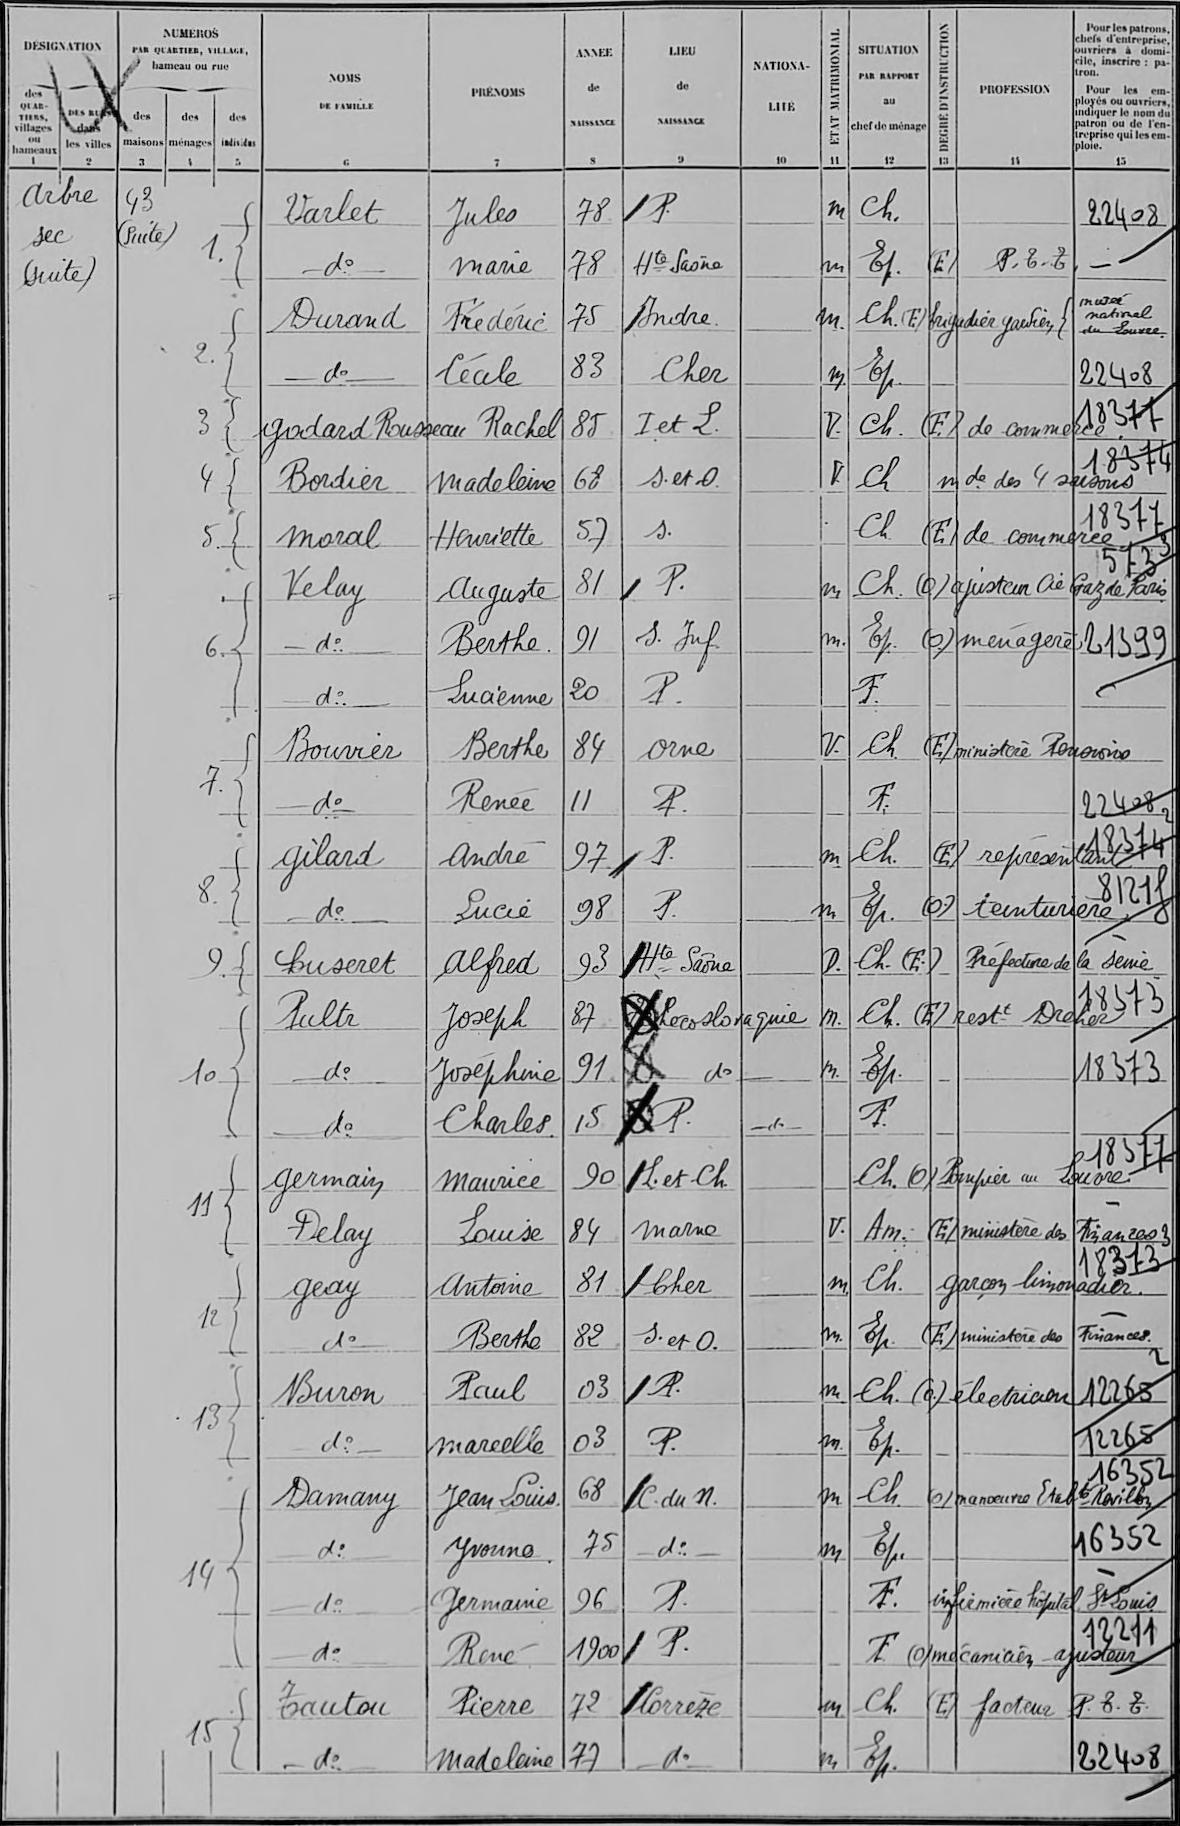Varlet/Jules/78/P/¤/M/Ch /¤/¤/22408
d°/marie/78/Hte Saône/¤/m/Ep/¤/(E) P T T /¤
Durand/Frédéric/75/Indre/¤/M/Ch /¤/(E) brigadier gardien/du Louvre?national?musée
d°/Cécile/83/Cher/¤/m/Ep/¤/¤/22408
Godard Rousseau/Rachel/85/I et L/¤/V/Ch/¤/(E) de commerce/?18377
Bordier/Madeleine/68/S et O /¤/V /Ch/¤/mde des 4 saisons/?18374
Moral/Henriette/57/S /¤/¤/Ch/¤/(E) de commerce/?18377
Velay/Auguste/81/P/¤/m/Ch/¤/(O) ajusteur cie Gaz de Paris/?573
d°/Berthe/91/S Inf/¤/m/Ep/¤/(o) ménagère/21399
d°/Lucienne/20/P/¤/¤/F/¤/¤/¤
Bouvier/Berthe/84/Orne/¤/V/Ch/¤/(E) ministère Pensions/¤
d°/Renée/11/P/¤/¤/F/¤/¤/22408
Gilard/André/97/P/¤/m/Ch/¤/(E) représentant/?18374
d°/Lucie/98/P/¤/m/Ep/¤/(o) teinturière/?8121f
Luseret/Alfred/93/Hte Saône/¤/D/Ch/¤/(E) Préfecture de la Seine/¤
Pultr/Joseph/87/Tchécoslovaquie/¤/m/Ch/¤/(E) restt Dreher/?18373
d°/Joséphine/91/d°/¤/m/Ep/¤/¤/18373
d°/Charles/15/P/d°/¤/F/¤/¤/¤
Germain/Maurice/90/L et Ch/¤/¤/Ch/¤/(O) Pompier au Louvre/?18377
Delay/Louise/84/marne/¤/V/Am/¤/(E) ministère des Finances/¤
Geay/Antoine/81/Cher/¤/m/Ch /¤/garçon limonadier/?18373
d°/Berthe/82/S et O/¤/m/Ep/¤/(E) ministère des Finances/¤
Buron/Paul/03/P/¤/m/Ch/¤/(O) éléctricien/12265
d°/marcelle/03/P/¤/m/Ep/¤/¤/12265
Damany/Jean louis/68/C du N/¤/m/Ch/¤/(O) manoeuvre étabts Ravillon/?16352
d°/Yvonne/75/d°/¤/m/Ep/¤/¤/16352
d°/Germaine/96/P/¤/¤/F/¤/infirmière hôpital St Louis/¤
d°/René/1900/P/¤/¤/F/¤/(O) mécanicien ajusteur/?72211
Tautou/Pierre/72/Corrèze/¤/m/Ch/¤/(E) facteur/P T T
d°/Madeleine/77/d°/¤/m/Ep/¤/¤/22408
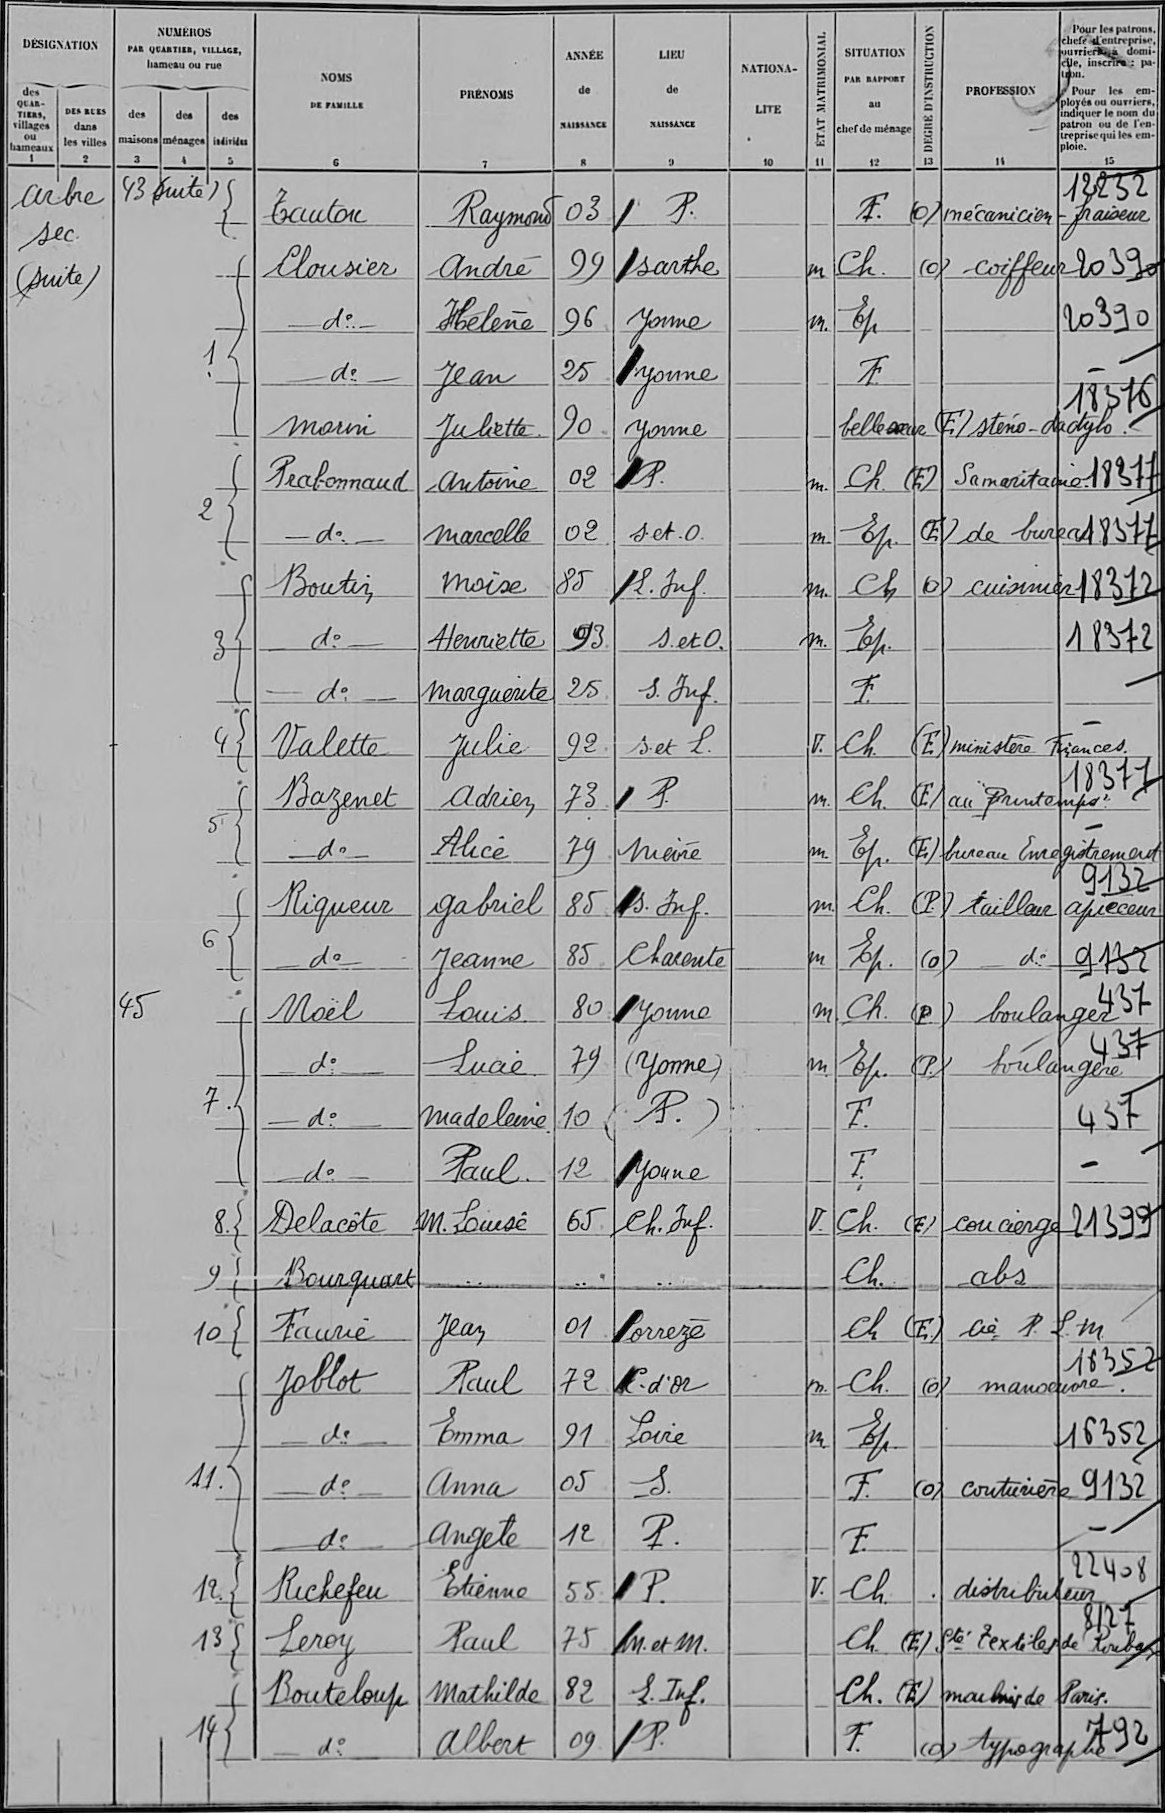Tautou/Raymond/03/P /¤/¤/F /¤/(o) mécanicien-fraiseur/12232
Clousier/André/99/sarthe/¤/m/Ch /¤/(o) coiffeur/20390
d°/Hélène/96/yonne/¤/m /Ep/¤/¤/20390
d°/Jean/25/yonne/¤/¤/F/¤/¤/¤
morin/Juliette/90/yonne/¤/¤/belle soeur/¤/(E) sténo-dactylo/?18376
Prabonnaud/Antoine/02/P/¤/m/Ch/¤/(E) Samaritaine/18377
d°/marcelle/02/s et o /¤/m/Ep/¤/(E) de bureau/18377
Boutin/moïse/85/L Inf/¤/m /Ch/¤/(O) cuisinier/18372
d°/Henriette/93/s et o /¤/m /Ep /¤/¤/18372
d°/marguerite/25/S Inf/¤/¤/F /¤/¤/¤
Valette/Julie/92/S et L /¤/V /Ch /¤/(E) ministère Finances/¤
Bazenet/Adrien/73/P/¤/m /Ch /¤/(E) au Printemps/?18377
d°/Alice/79/Nièvre/¤/m/Ep /¤/(E) bureau Enregistrement/¤
Riqueur/Gabriel/85/S Inf /¤/m/Ch /¤/(P) tailleur apiéceur/?9132
d°/Jeanne/85/Charente/¤/m/Ep /¤/(o) tailleur apiéceur/?9132
Noël/Louis/80/yonne/¤/m/Ch /¤/(P) boulanger/?437
d°/Lucie/79/(yonne)/¤/m/Ep /¤/(P) boulangère/?437
d°/madeleine/10/(P )/¤/¤/F /¤/¤/437
d°/Paul/12/yonne/¤/¤/F /¤/¤/¤
Delacôte/M Louise/65/Ch Inf /¤/V /Ch /¤/(E) concierge/21399
Bourquart/¤/¤/¤/¤/¤/Ch /¤/abs/¤
Faurie/Jean/01/Corrèze/¤/¤/Ch/¤/(E) cie P L M /¤
Joblot/Paul/72/C d'Or/¤/m/Ch /¤/(o) manoeuvre/?16352
d°/Emma/91/Loire/¤/m/Ep/¤/¤/16352
d°/Anna/05/S /¤/¤/F /¤/(o) couturière/9132
d°/Angèle/12/P /¤/¤/F /¤/¤/¤
Richefeu/Etienne/55/P /¤/V /Ch/¤/distributeur/?22408
Leroy/Paul/75/M et M /¤/¤/Ch /¤/(E) Sté textiles de Roubaix/?8127
Bouteloup/Mathilde/82/L Inf /¤/¤/Ch /¤/(E) machines de Paris/¤
d°/Albert/09/P /¤/¤/F /¤/(o) typographe/?792